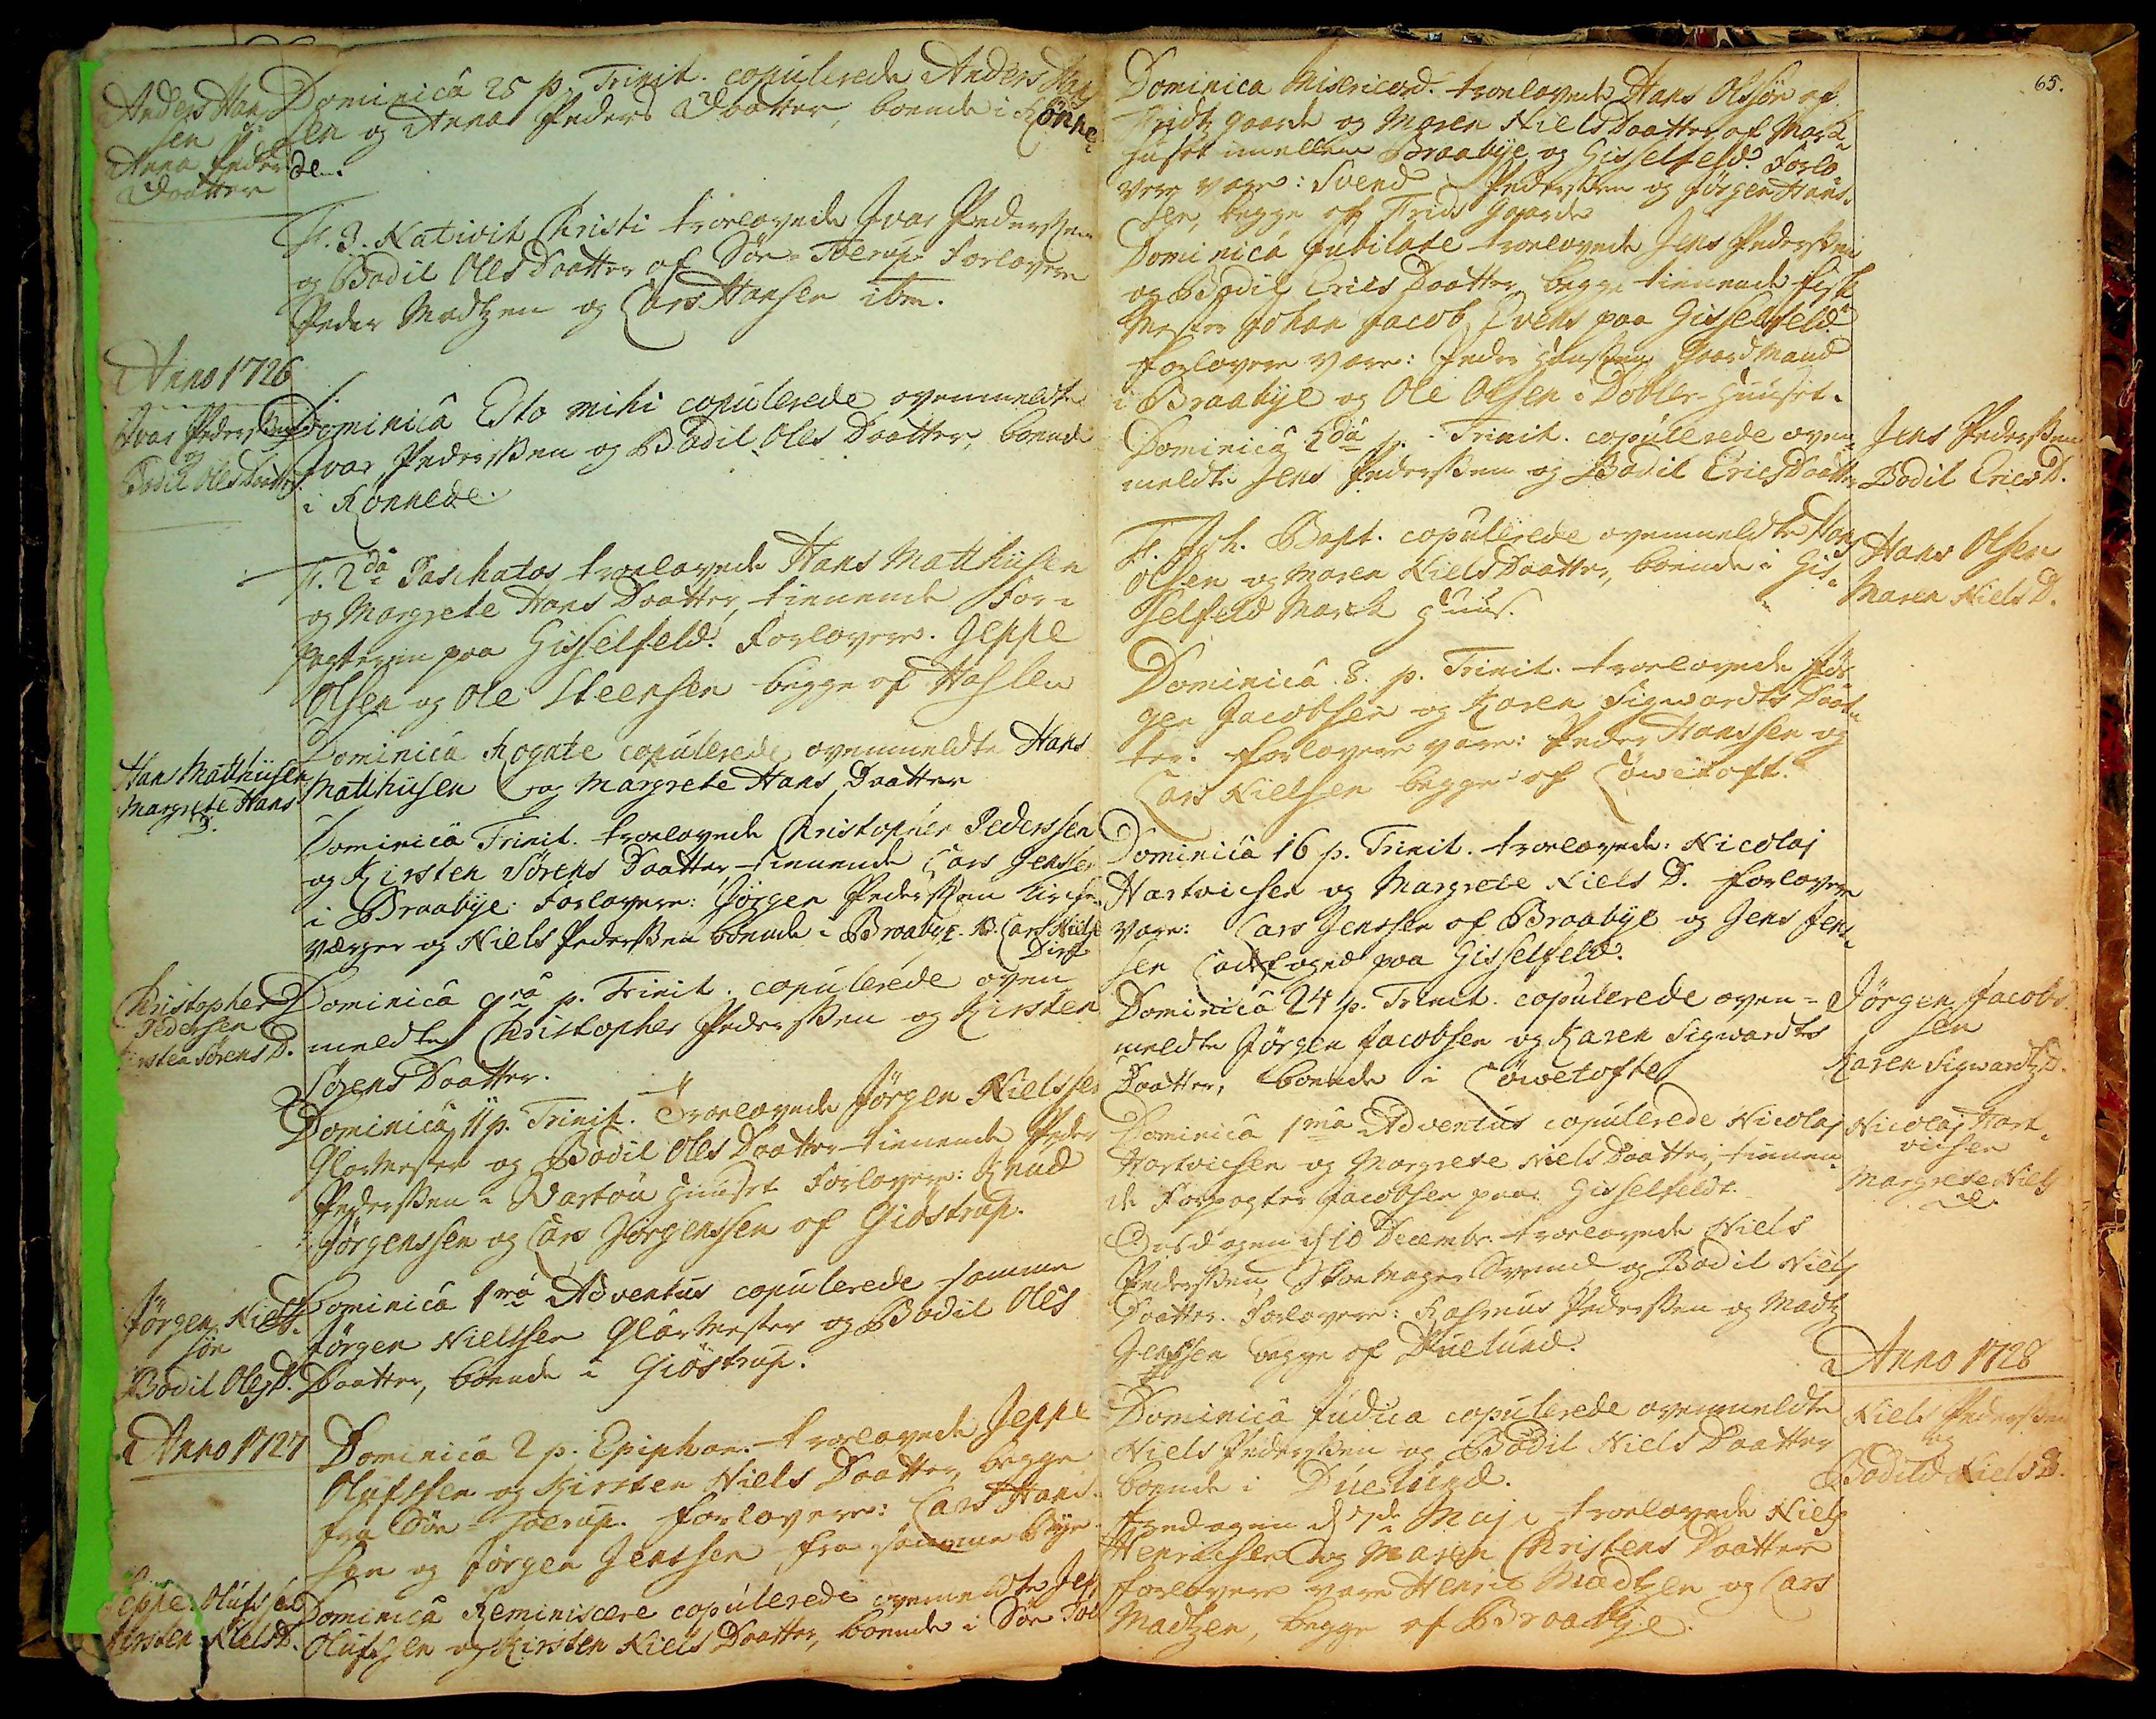Anders Hans¬
sen 
Anna Peders
Daatter 
Dominica 25 p. Trinit. copulerede Anders Hans
sen og Anna Peders Daatter, boende i Rønne¬
de.
F3. Nativit Christi troelovede Ivar Pedersen 
og Bodil Oles Daatter af Søe Toerup. Forlovere: 
Peder Madtzen og Lars Hansen ibm.
Anno 1726 
Ivar Pederssen 
og
Bodil OlesDaatter 
Dominica Esto mihi copulerede ovenmeldte 
Ivar Pederssen og Bodil Oles Daatter, boende 
i Rønnede. 
F. 2da Paschatos troelovede Hans Matthiisen
og Margrete Hans Daatter, tienende For¬
pagteren paa Gisselfeld. Forlovere: Jeppe
Olsen og Ole Steensen begge af Hasle.
Hans Matthiisen 
Margrete Hans
D. 
Dominica Rogate copulerede ovenmeldte Hans
Matthiisen og Margrete Hans Daatter. 
Dominica Trinit. troelovede Christopher Pederssen
og Kirsten Sørens Daatter tienende Lars Jensen
i Braabye. Forlovere: Jørgen Pederssen Kirche¬
værge og Niels Pederssen boende i Braabye NB.## Lars Nielsen
Dire##
Christopher
Pedersen
Kirsten Sørens D.
Dominica 9ne p. Trinit. copulerede oven
meldte Christopher Pederssen og Kirsten 
Sørens Daatter.
Dominica 11.p. Trinit. Troelovede Jørgen Nielssen
Glarmester## og Bodil Oles Daatter tienende Peder
Pederssen i Wartou Huuset, Forlovere: Knud
Jørgenssen og Lars Jørgenssen af Giøstrup.
Jørgen Niels¬
søn
Bodil OlesD.
Dominica 1ma Adventus copulerede samme
Jørgen Nielssen Glarmester## og Bodil Oles
Daatter, boende i Giøstrup. 
Anno 1727  
Dominica 2. p. Epiphan troelovede Jeppe
Olufssen og Kirsten Niels Daatter, begge
fra Søe Toerup. Frolovere: Lars Hans¬
sen og Jørgen Jenssen fra samme Bye.
eppe## Olufssen
Kirsten NielsD.
Dominica Reminiscere copulerede ovenmeldte Jep
Olufssen og Kirsten Niels Daatter, boende i Søe Toe 
Dominica Misericord. troelovede Hans Olssøn af
Fridtzgaard og Maren Niels Daatter af Mark¬
huset imellem Braabye og Gisselfeld. Forlo¬
vere vare: Svend Pederssen og Jørgen Hans¬
sen, begge af Fridsgaarde.
65.
Dominica Jubilate troelovede Jens Pederssen
og Bodil Erics Daatter begge tienende Fiske
mester Johan Jacob Evens paa Gisselfeld.
Forlovere vare: Peder Hanssen, Gaardmand
i Braabye og Ole Olsen i Dobler-huuset.
Dominica 2da p. Trinit. copulerede oven¬
meldte Jens Pederssen og Bodil Erics Daatter. 
Jens Pederssen 
Bodil EricsD.
F. Joh copulerede ovenmeldte Hans 
Olsen og Maren Niels Daatter, boende i Gis¬
selfeld Mark Huus.
Hans Olsen
Maren NielsD.
Dominica 8.p.Trinit. troelovede Jør¬
gen Jacobsen og Karen Sigvardts Daat¬
ter. Forlovere vare: Peder Hanssen og
Lars Nielsen, begge af Løvetoft.
Dominica 16.p. Trinit. troelovede Nicolaj
Hartvicsen og Margrete Niels D. Forlovere
vare: Lars Jenssen af Braabye og Jens Jens¬
sen Ladefoged paa Gisselfeld. 
Dominica 24 p. Trinit. copulerede oven¬
meldte Jørgen Jacobsen og Karen Sigvardts
Daatter, boende i Løvetofte.
Jørgen Jacobs¬
sen
Karen SigvardtzD.
Dominica 1ma Adventus copulerede Nicolaj
Hartvicsen og Margrete Niels Datter, tienen¬
de Forpagter Jacobsen paa Gisselfeldt.
Nicolaj Hart¬
vicsen
Margrete Niels
D.
Onsdagen d. 10 Decembr. troelovede Niels
Pederssen Skoemager Svend og Bodil Niels
Daatter. Forlovere: Rasmus Pederssen og Madtz
Jenssen, begge af Duelund.
Dominica Judica copulerede ovenmeldte
Niels Pederssen og Bodil Niels Daatter,
boende i Duelund.
Anno 1728
Niels Pederssen 
og 
Bodild NielsD.
Fredagen d. 7de Maj troelovede Niels
Henricsen og Maren Christens Datter.
Forlovere vare: Henric Madtzen og Lars
Madtzen, begge af Braabye.